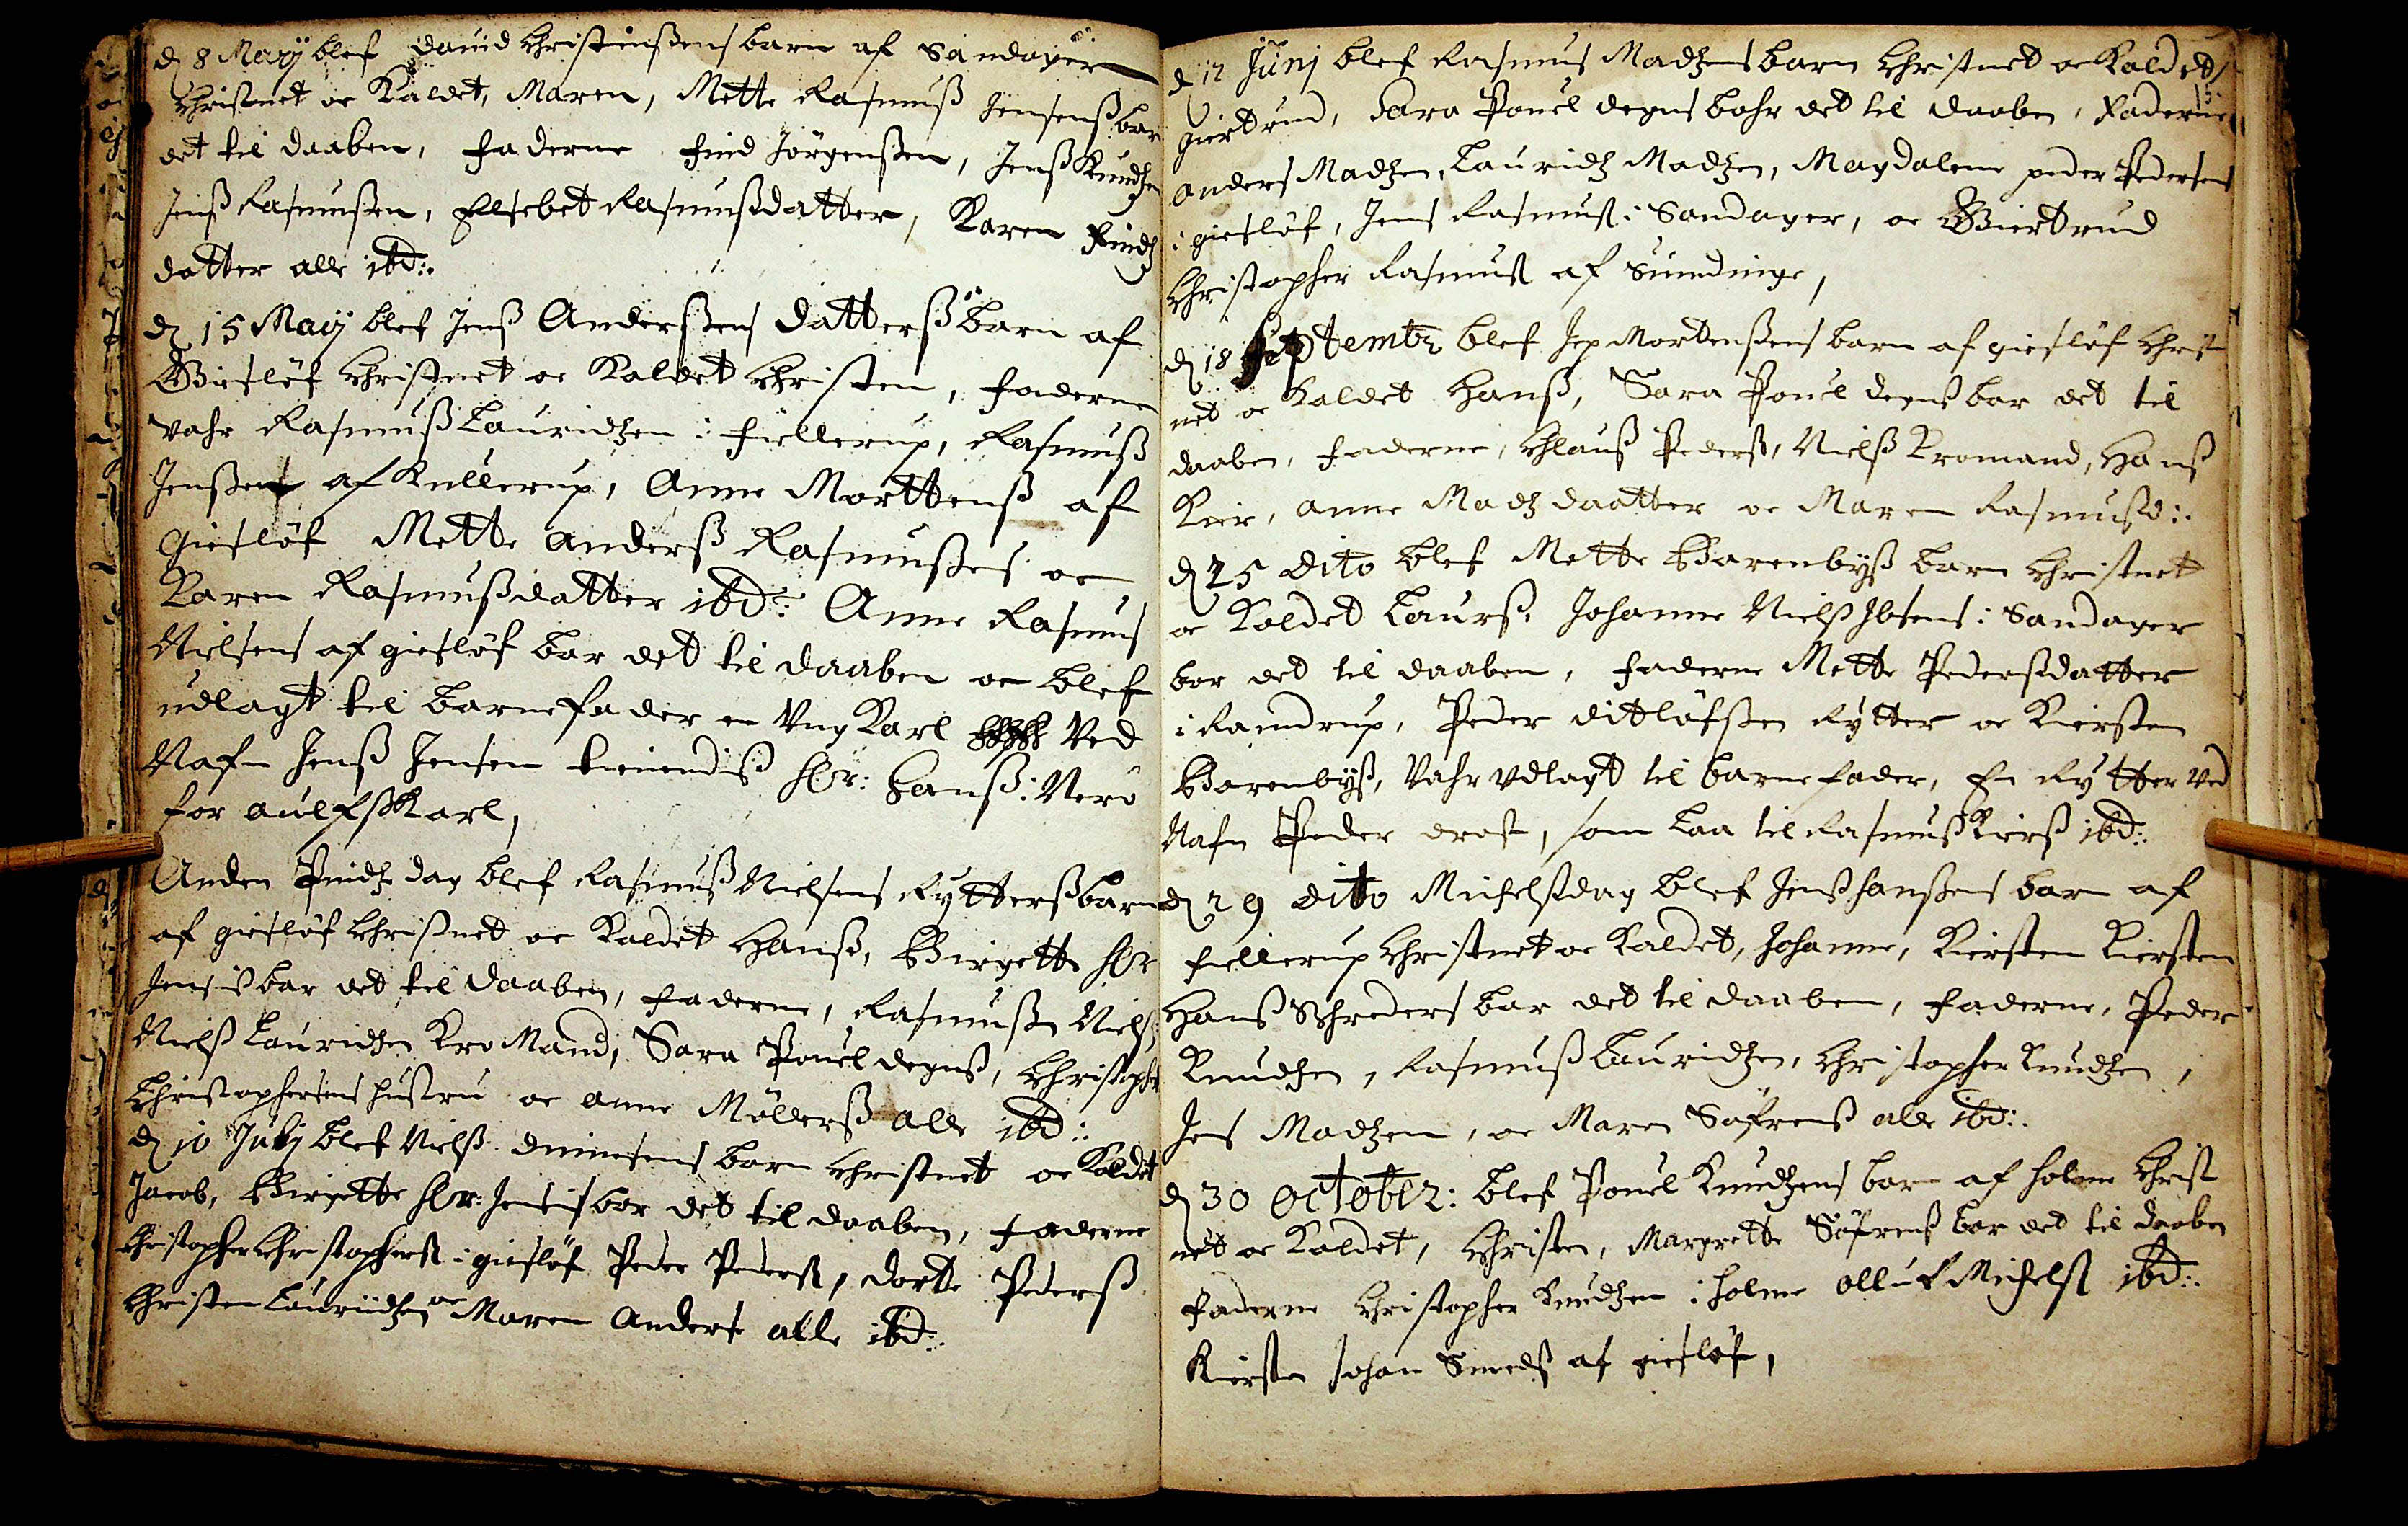d 8 Maij blef Dauid Christenssens barn af Samdager
Christnet oc kaldet Maren, Mette Rasmuss Jensenss bar
det til daaben, Faderne Find Jørgenssen, Jenss Knudtzen
Jenss Rasmussen, Elsebet Rasmussdatter, Karen Findtz
datter alle ibd:.
D 15 Maij blef Jenss Anderssens datterss barn af
Giesløf Christenet oc Kalvet Christen, Faderne
vahr Rasmuss Lauridtzen i Fiellerup, Rasmuss
Jenssen af Kullerup, Anne Morttenss af
Giesløf Mette Anderss Rasmussens oc
Karen Rasmussdatter ibd: Anne Rasmus
Nielsens af giesløf bar det til daaben oc blef
udlagt til barnefader en vng karl ## ved
Nafn Jenss Jensen tienendis hlr: Hanss
for aulfsskarl,
Anden Prndtz dag blef Rasimuss Nielsens Rytterss barn
af giesløf Christnet oc kaldet Hanss, Birgette hlr
Jensis bar det til daaben, Faderene, Rasmuss Nielss
Nielss Lauridtzen Kro Mand; Sara Pouel Degnss, Christopher
Christophersens Hustru oc Anne Møllerss alle ibd:.
d 10 Julij blef Nielss Dinnesens barn Cristnet oc kaldet
Jacob, Birgette hlr: Jensis bar det til daaben, Faderene
Christopher Christophersenss i giesløf Peder Pederss, Dorte Pederss
oc
Christen Lauridtzen Maren Anders alle ibd:
15.
d 12 Junj blef Rasmus Madtzens barn Christnet oc kaldet
giertrud, Sara Pouel degns bahr det til daaben, Faderne
Anders Madtzen Lauridtz Madtzen, Magdalene peder Pedersens
i giesløf, Jens Rasmuss i Sandager, oc Giertrud
Christopher Rasmuss af Suindinge
d 18 Septembr blef Jep Mortenssens barn af gieslef Christ
net oc kaldet Hanss, Sara Pouel degnss bar det til
daaben, Faderne, Chlauss Pederss, Nielss Kromand, Hanss
Kier, Anne Madtzdaatter oc Maren Rasmusd:
d 25 Dito blef Mette Barenbyss barn Christnet
oc kaldet Laurss. Johanne Nielss Ibsens i  Sandager
bar det til daaben, Faderne Mette Pederssdatter
i Ramdrup, Peder Ditløfssen Rytter oc Kiersten
Barenbyss, Vahr vdlagt til barnefader, Enr Rytter ved
Nafn Peder drost, som laa til Rasmuss Kierss ibd:.
d 29 Dito Michelsdag blef Jenss Hanssen barn af
fiellerup Christnet oc kaldet, Johanne, Kiersten Kiersten
Hans Schreders bar det til daaben, Faderne, Peder
Knudtzen, Rasmuss Lauridtzen, Christopher Knudtzen
Jens Madtzen, oc Maren Søfrens alle ibd:.
d 30 Octobr: blef Pouel Knudtzens barn af Holme Christ¬
net oc kaldet, Christen, Margrette Søfrens bar det til daaben
Faderne Christopher Knudtzen i holme Olluf Michels ibd:.
Kiersten Johan Smeds af giesløf,
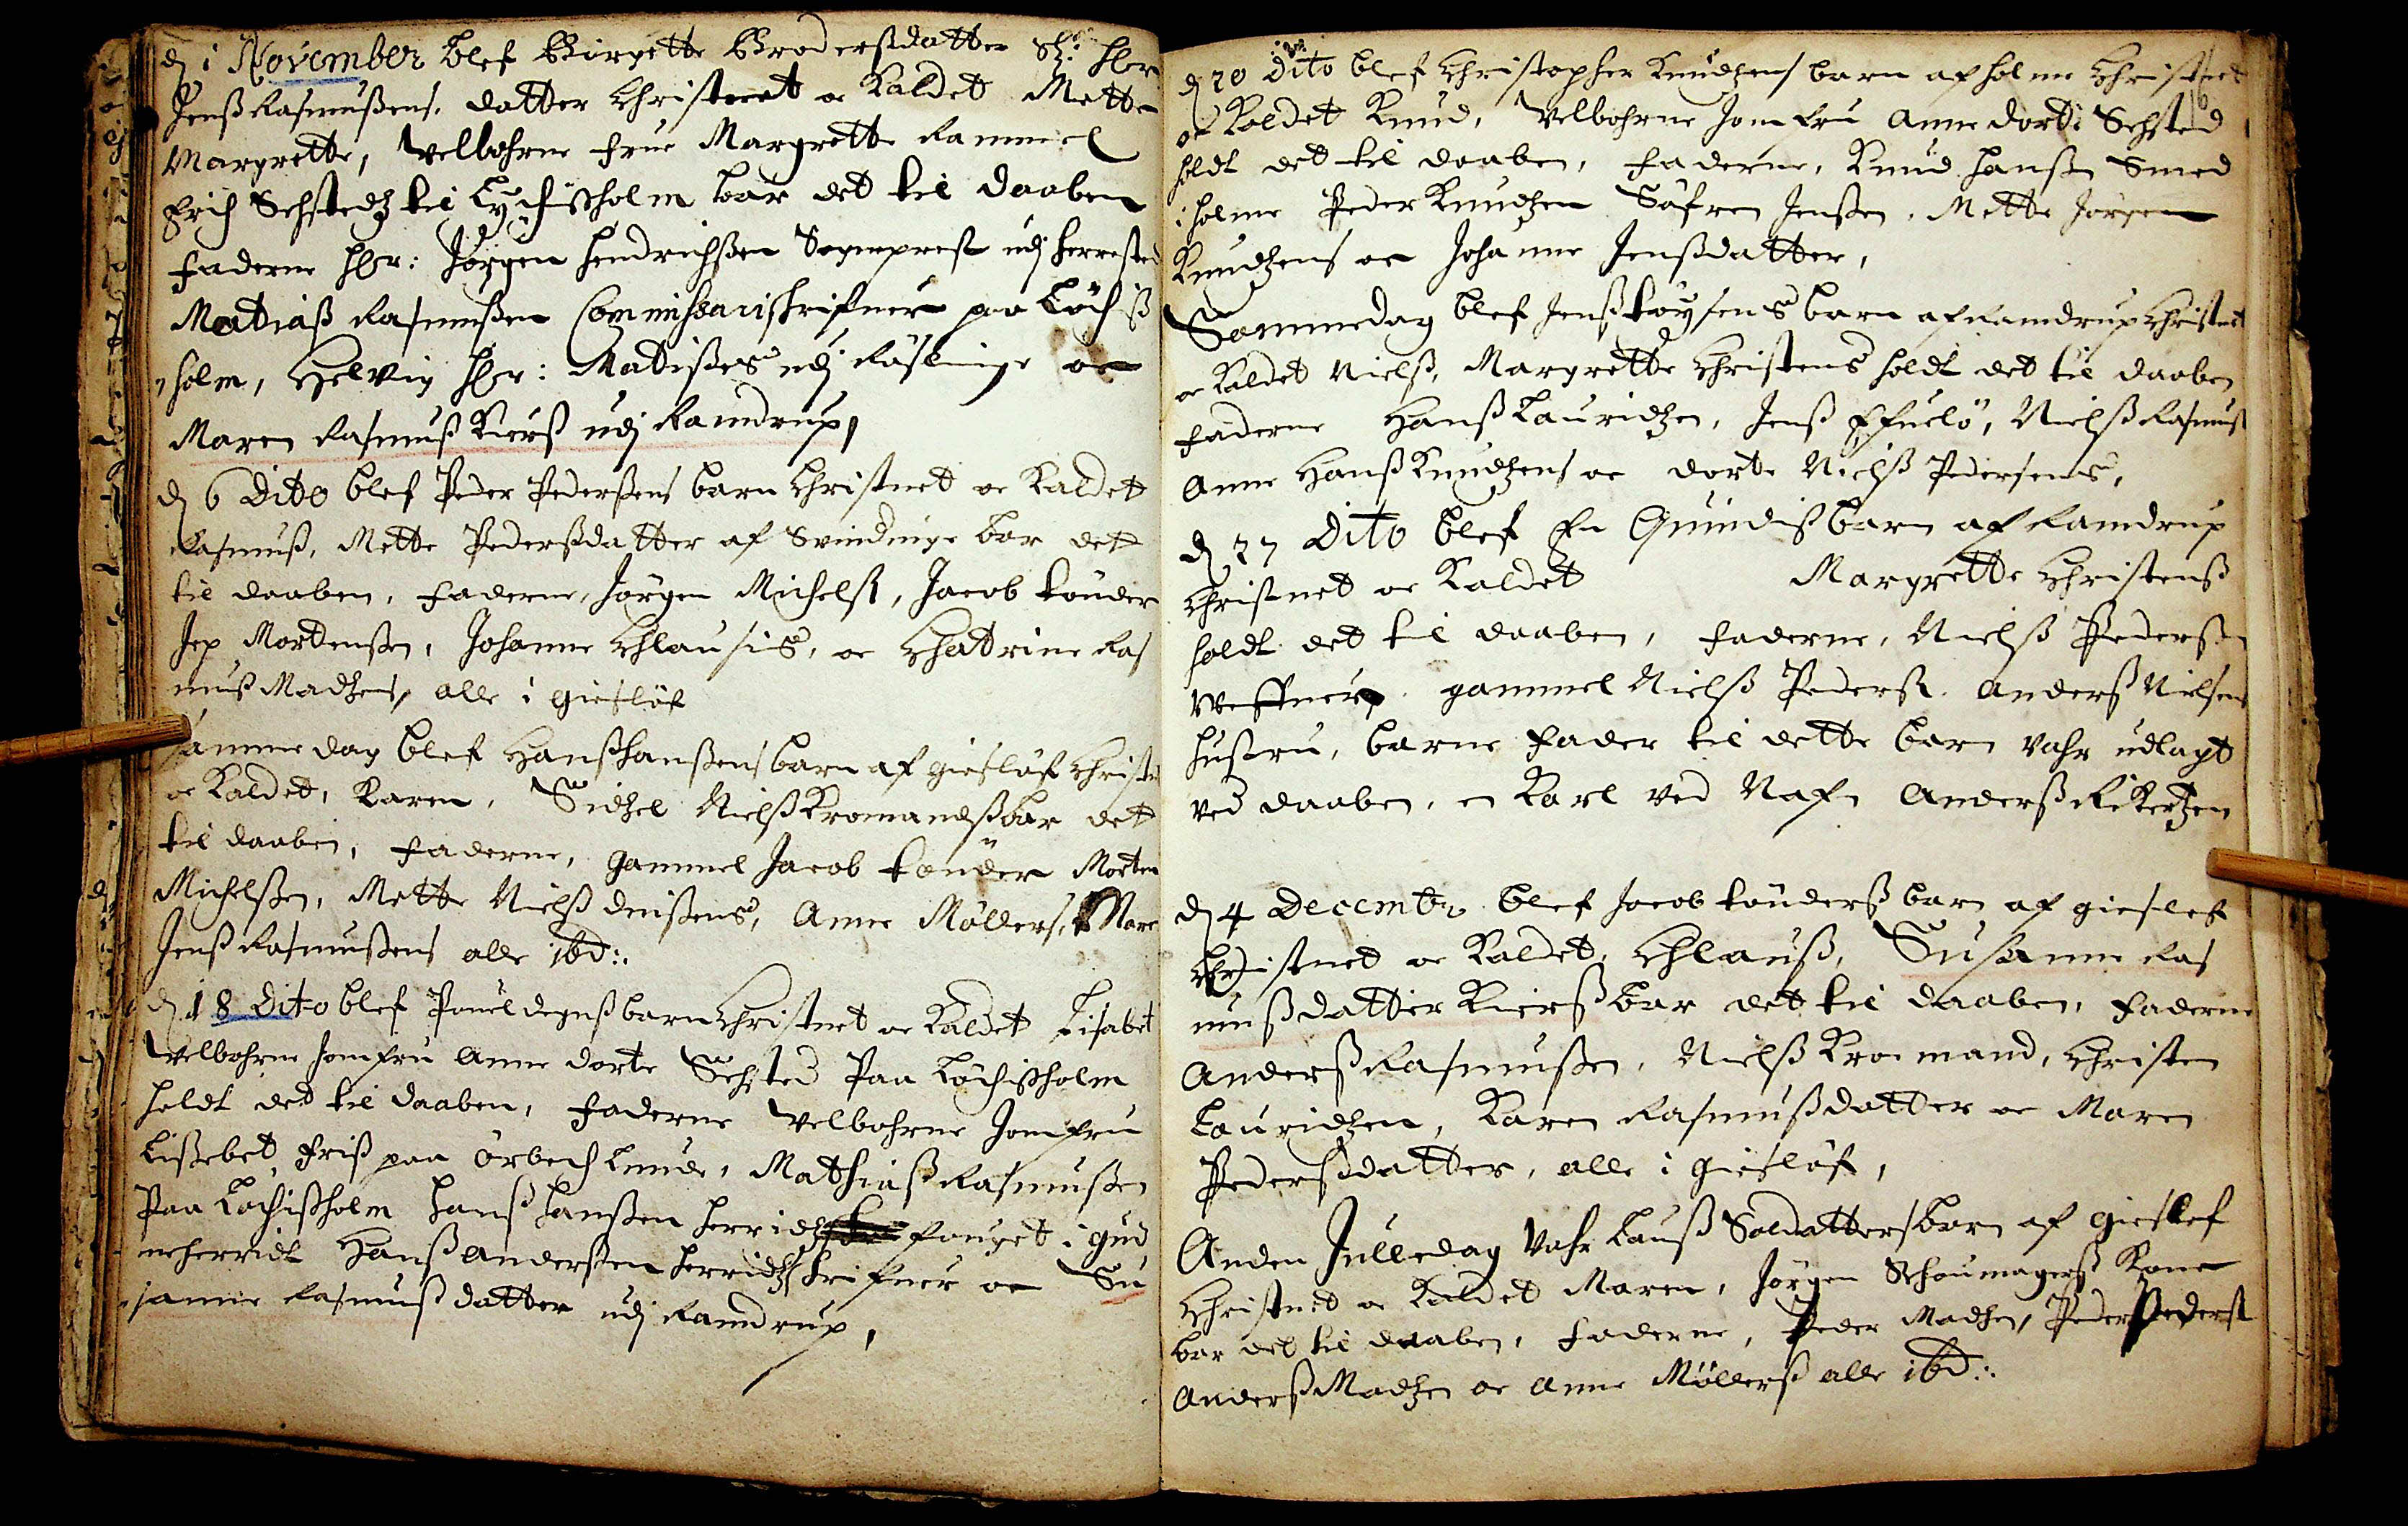d 1 November blef Birrette Broderssdatter Sl: hlr
Jens Rasmussens. datter Christenet oc kaldet Mette
Margrette, Velbohrne frue Margrette Rammel
Erich Sehstedtz til Lychisholm bar det til Daaben
Faderene hlr: Jørgen Hendrichssen Sogneprest udi Herreste
Mattias Rasmussen Commihariskrifuer paa Løchiss
= holm, Helving hlr: Mattisses udi Røsslinge oc
Maren Rasmuss Kierss udi Ramdrup,
d 6 Dito blef Peder Pederssens barn Christnet oc kaldet
Rasmus. Mette Pederssdatter af Svindinge bar det
til daaben, Faderne, Jørgen Michels, Jacob Tønder
Jep Mortenssen, Johanne Chlausis, oc Chatrine Ras
muss Madtzes, alle i Giesløf
samme dag blef Hanss Hanssens barn af Giesløf Christn
oc kaldet, Karen, Sidtzel Nielss Kromandss bar det
til daaben, Faderne, Gammel Jacob Tønder Morten
Michelssen, Mette Nielss Dinssens, Anne Møllers ##nr
Jenss Rasmusses alle ibd:.
d 18 Dito blef Pouel degnss barn Christenet oc kaldet Lisabet
Velbohrne Jomfru Anne dorte Sehsted Paa Løchisholm
holdt det til daaben, Faderne Velbohrne Jomfru
Lissebet Friss paa Ørbechlund, Mathiass Rasmussen
Paa Løchissholm Hanss Hanssen Herridtz fouget i Gud
meherridt Hanss Anderssen Herridtz Hrfuer oc Su
= sanne Rassmusdatter udi Randrup,
16
d 20 Dito bleff Christopher Knudtzens barn af holme Christnet
oc kaldet Knud, Velbohrne Jomfru Anne Dorte Sehsted
holdt det til daaben, Faderne, Knud Hanssen Smed
i Holme Peder Knudtzen Søfren Jenssen, Mette Jørgen
Knudtzens oc Johanne Jenssdatter,
Sammedag blef Jenss tøyysens barn af Ramdrup Christnet
oc kaldet Niels, Margrette Christens holdt det til daaben
Faderne Hans Lauridtzen, Jenss Efuelø, Niels Rasmus
Anne Hans Knudtzens oc Dorte Niels Pedersens
d 27 Dito blef En Gunidiss barn af Ramdrup
Christnet oc kaldet
Margrette Christenss
holdt det til daaben, Faderne, Nielss Pederssen
gammel Nielss Peders. Anderss Nielsens
Hustru, barne fader til dette barn vahr udlagt
ved daaben, en karl ved Nafn Anderss Rikertzen
d 4 Decembr blef Jacob tønderss barn af Gieslef
Christnet oc kaldet Chlauss, Susamme Ras
mussdatter Kierss bar det til daaben, Faderne
Anderss Rasmussen, Niels Kro mand, Christen
Lauridtzen, Karen Rasmussdatter oc Maren
Pederssdatter, alle i Giesløf,
Anden Julledag Vahr Lauss Soldatters barn af Gieslef
Christnet oc kaldet Maren, Jørgen Schoumagerss Kone
bar det til daaben, Faderne, Peder Madtzen, Peder Peders
Anderss Madtzen oc Anne Møllerss alle ibd:.